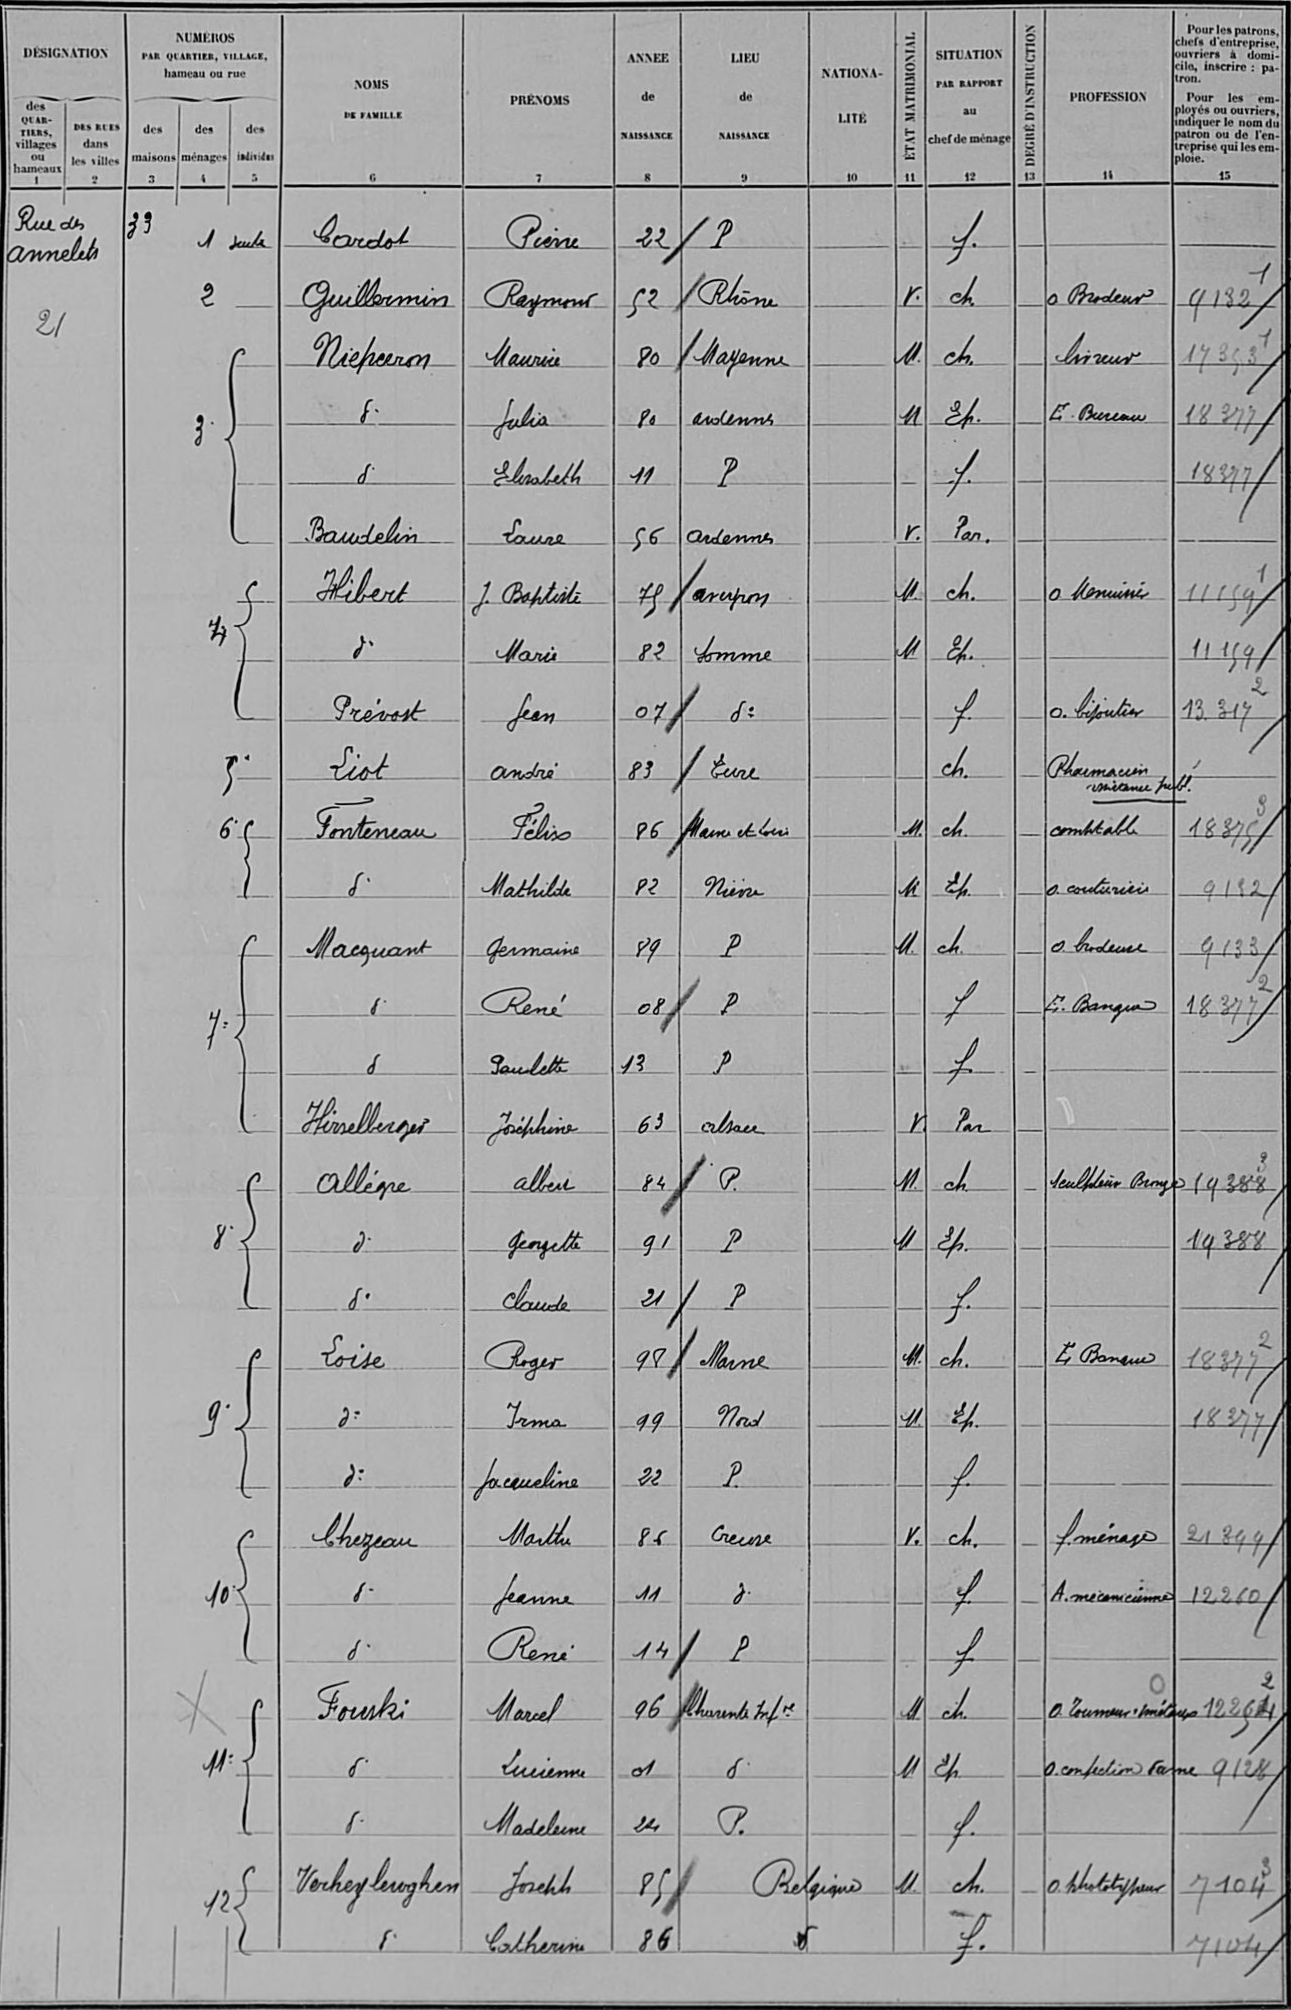Bardot/Pierre/22/P/¤/¤/f /¤/¤/¤
Guillermin/Raymond/52/Rhône/¤/V /ch/¤/o Brodeur/9132
Nieheeron/Maurice/80/Mayenne/¤/M /ch /¤/livreur/17343
d°/Julia/80/ardennes/¤/M/Ep /¤/E Bureau/18377
d°/Elisabeth/11/P/¤/¤/f /¤/¤/18377
Baudelin/Laure/56/ardennes/¤/V /par /¤/¤/¤
Hilbert/J Baptiste/75/Aveyron/¤/M/ch /¤/o Menuisier/11159
d°/Marie/82/Somme/¤/M/Ep /¤/¤/11159
Prévost/jean/07/d°/¤/¤/f /¤/o bijoutier/13317
Liot/andré/83/Eure/¤/¤/ch /¤/Pharmacien!assistance publ /¤
Fonteneau/Félix/86/Maine et Loire/¤/M/ch /¤/comptable/18375
d°/Mathilde/82/Nièvre/¤/M/Ep /¤/o couturiere/9132
Macquant/Germaine/89/P/¤/M/ch /¤/o brodeuse/9133
d°/René/08/P/¤/¤/f/¤/E banque/18377
d/Paulette/13/P/¤/¤/f/¤/¤/¤
Hirselberger/Joséphine/63/alsace/¤/V /Par/¤/¤/¤
Allégre/albert/84/P /¤/M /ch/¤/sculpteur bronze/19388
d°/Georgette/91/P/¤/M/Ep /¤/¤/19388
d°/Claude/21/P/¤/¤/f /¤/¤/¤
Loise/Roger/98/Marne/¤/M /ch /¤/E Banque/18377
d°/Irma/99/Nord/¤/M /Ep /¤/¤/18377
d°Jacqueline/22/P /¤/¤/f /¤/¤/¤/¤
Chezeau/Marthe/86/Creuse/¤/V /ch /¤/f ménage/21399
d°/Jeanne/11/d°/¤/¤/f/¤/A Mecanicienne/12260
d°/René/14/P/¤/¤/f/¤/¤/¤
Fourki/Marcel/96/Charente Infre/¤/M/ch /¤/O tourneur métaux/12264
d°/Lucienne/01/d°/¤/M/Ep/¤/O confection fame/9128
d°/Madeleine/24/P /¤/¤/f /¤/¤/¤
Verheylewghen/Joseph/85/Belgique/¤/M /ch /¤/O phototypeur/7104
d°/Catherine/86/d°/¤/¤/f /¤/¤/7104
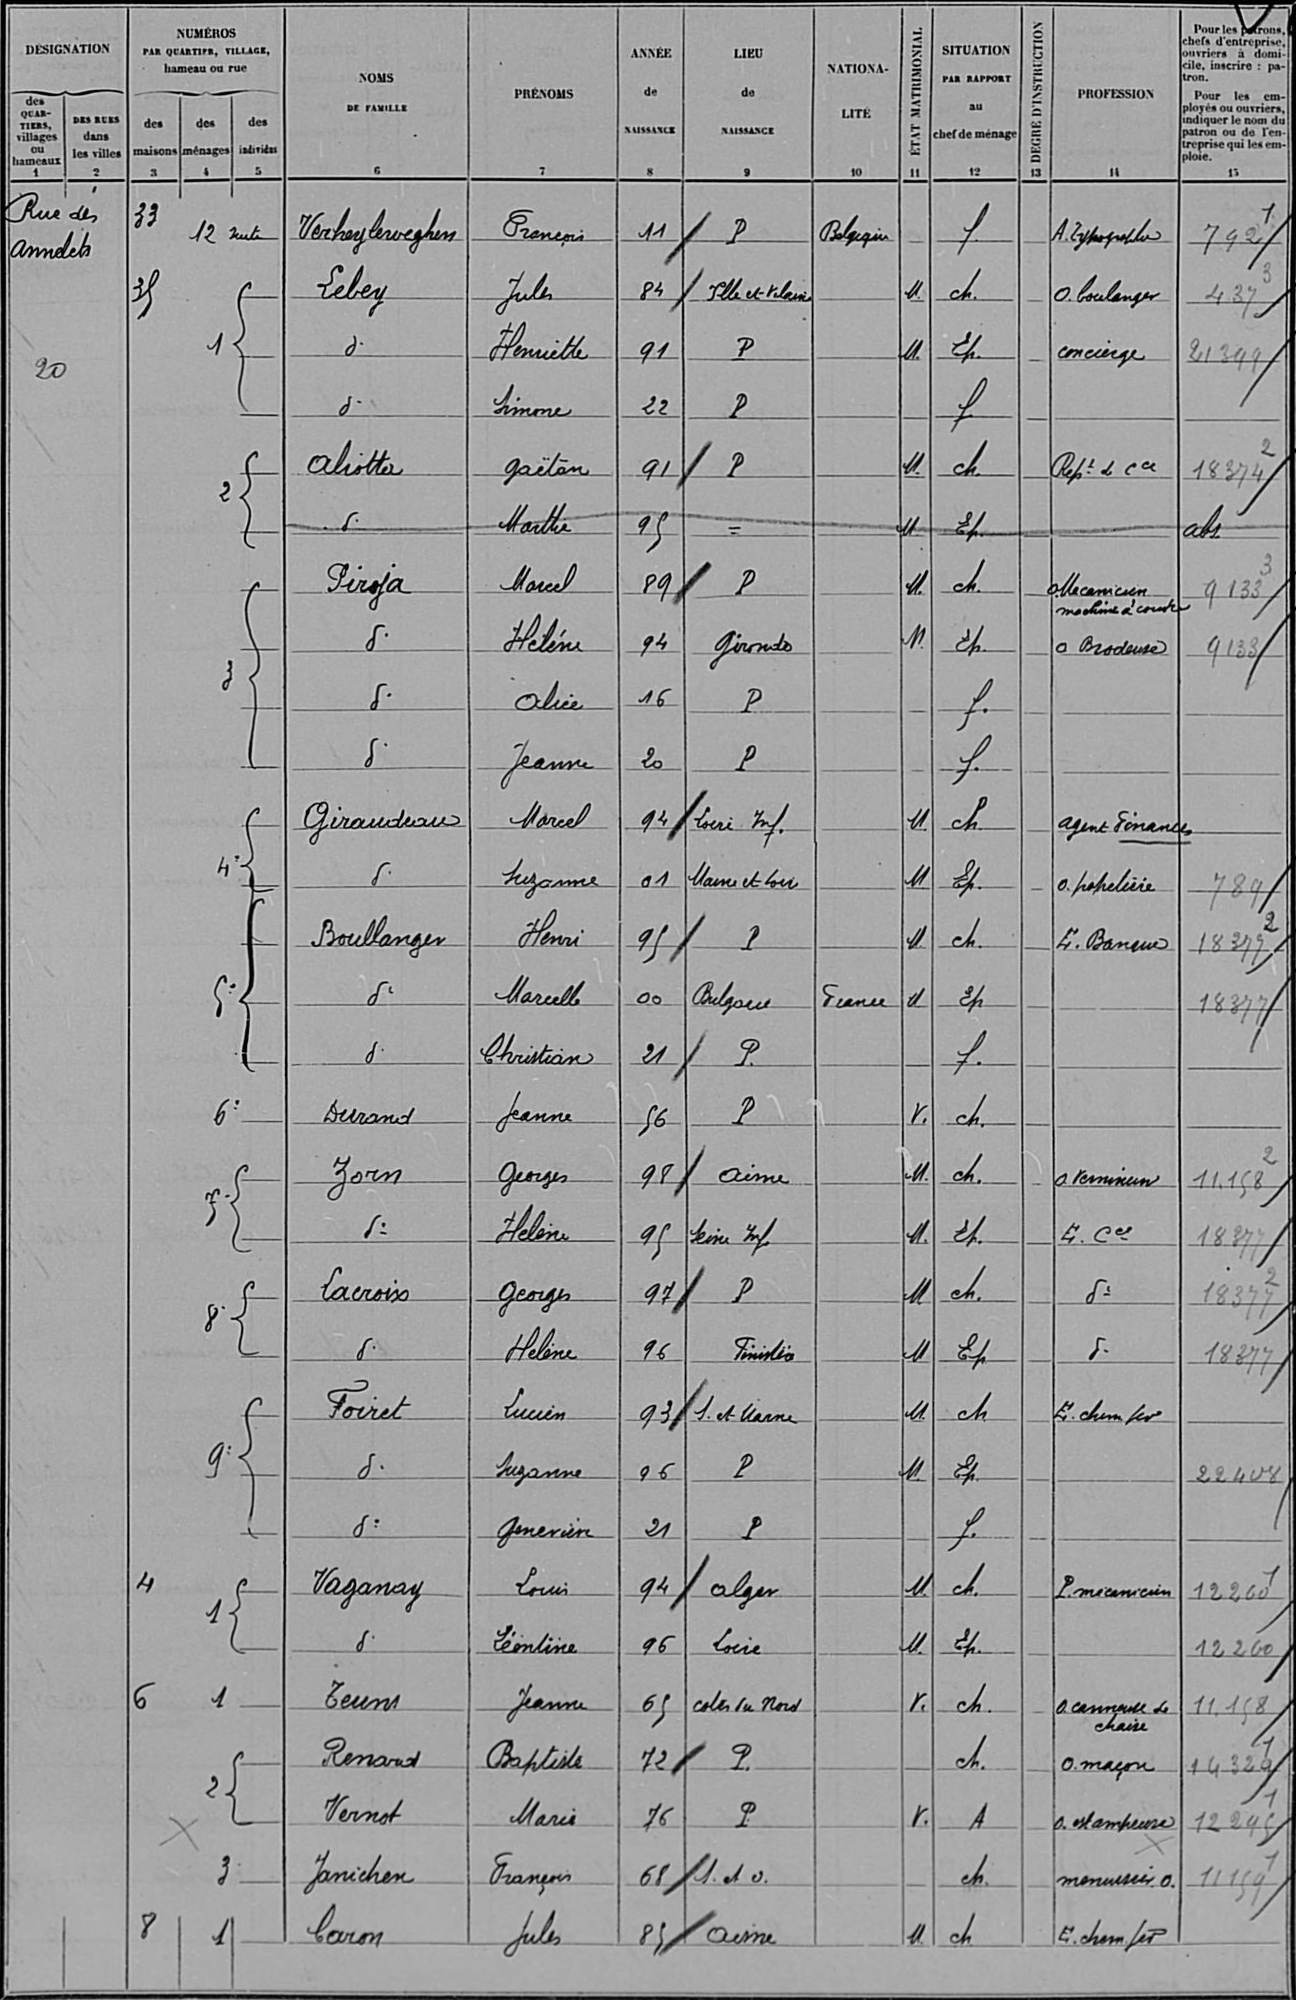Verheyleweghen /Francois/11/P/Belgique/¤/f/¤/A Typographe/742
Lebey/Jules/84/Ille-et-Vilaine/¤/M/ch /¤/O boulanger/437
d /Henriette/91/P/¤/M/Ep /¤/concierge/21399
d /Simone/22/P/¤/¤/f/¤/¤/¤
Abioter/Gaëtan/91/P/¤/M/ch /¤/Rep de Cce/18374
d°/Marthe/95/¤/¤/M/Ep /¤/¤/abs
Piroja/Marcel/89/P/¤/M/ch /¤/Mecanicien!machine à coudre/9133
d°/Hélène/94/Gironde/¤/M/ch /¤/o Brodeuse/9133
d°/Alice/16/P/¤/¤/f /¤/¤/¤
d°/Jeanne/20/P/¤/¤/f /¤/¤/¤
Giraudeau/Marcel/94/Loire Inf /¤/M /ch /¤/agent finances/¤
d°/Suzanne/01/Maine et Loir/¤/M/Ep /¤/o papetière/789
Boullanger/Henri/95/P/¤/M/ch /¤/E Banque/18377
d°/Marcelle/00/Bulgare/France/d/Ep/¤/¤/18377
d°/Christian/21/P /¤/¤/f /¤/¤/¤
Durand/Jeanne/56/P/¤/V /ch /¤/¤/¤
Zorn/Georges/98/Aisne/¤/M /ch /¤/o vernisseur/11158
d°/Hélène/95/Seine Inf/¤/M /Ep /¤/E Cce/18377
Lacroix/Georges/97/P/¤/M/ch /¤/d°/18377
d°/Helène/96/Finistère/¤/M/Ep /¤/d°/18377
Foiret/Lucien/93/s et Marne/¤/M/ch/¤/E chem fer/¤
d°/Suzanne/96/P/¤/M/Ep/¤/¤/22408
d°/Geneviève/21/P/¤/¤/f /¤/¤/¤
Vaganay/Louis/94/Alger/¤/M/ch /¤/P mecanicien/12260
d°/Léontine/96/Loire/¤/M /Ep /¤/¤/12260
Zeuns/Jeanne/65/cotes du Nord/¤/V /ch /¤/O canneleur de chaise/11158
Renaud/Baptiste/72/P /¤/¤/ch /¤/O Maçon/14329
Vernot/Marie/76/P/¤/V /A/¤/o estampeuse/12245
Janichen/François/68/s et o /¤/¤/ch /¤/menuisier o /12159
Caron/Jules/85/aisne/¤/M /ch/¤/E chem fer/¤